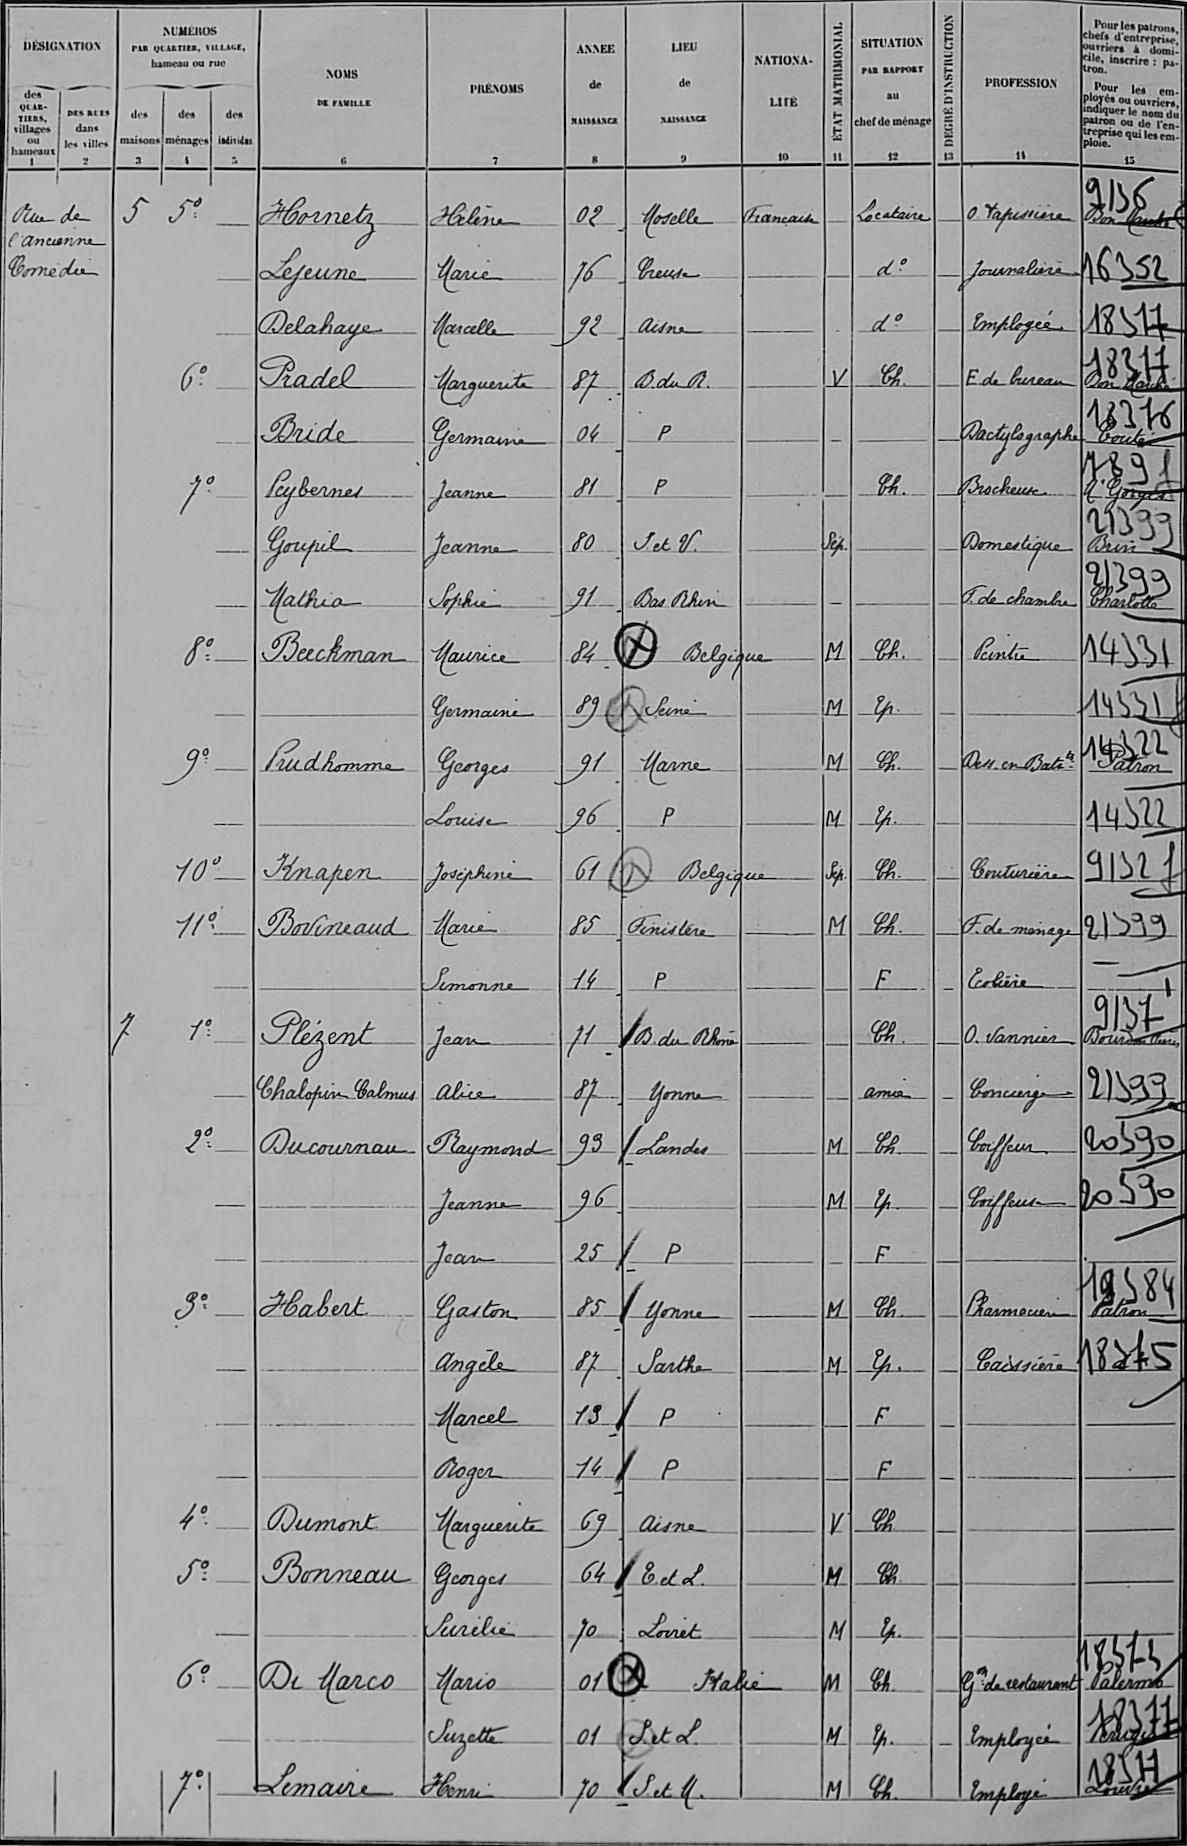Hornetz/Hélène/02/Moselle/française/¤/Locataire/¤/O Tapissière/Bon Marché?9136
Lejeune/Marie/76/Creuse/¤/¤/d°/¤/Journalière/16352
Delahaye/MArcelle/92/Aisne/¤/¤/d°/¤/Employée/18377
Pradel/Marguerite/87/B du R /¤/V/Ch /¤/E de bureau/Bon Marché?18377
Bride/Germaine/04/P/¤/¤/¤/¤/Dactylographe/Couté?18376
Peybernes/Jeanne/81/P/¤/¤/Ch /¤/Brocheuse/M Georges?7891
Goupil/Jeanne/80/I et V /¤/Sép/¤/¤/Domestique/Brin?21399
Mathia/Sophie/91/BAs Rhin/¤/¤/¤/¤/F de chambre/Charlotte?21399
Berckman/Maurice/84/Belgique/¤/M/Ch /¤/Peintre/14331
¤/Germaine/89/Seine/¤/M/Ep /¤/¤/15331
Prudhomme/geroges/91/Marne/¤/M/Ch /¤/Dess en Batx/Patron?14322
¤/Louise/96/P/¤/M/Ep /¤/¤/14322
Knapen/Joséphine/61/Belgique/¤/Sep /Ch /¤/Couturière/91327
Bovineaud/Marie/85/Finistère/¤/M/Ch /¤/F de méange/21399
¤/Simonne/14/P/¤/¤/F/¤/Ecolière/¤
Plézent/Jean/71/B du Rhône/¤/¤/Ch /¤/O vannier/Bourdon frères?9137
Chalopon Calmus/Alice/87/Yonne/¤/¤/amie/¤/Concierge/21399
Ducournau/Raymond/93/Landes/¤/M/Ch /¤/Coiffeur/20390
¤/Jeanne/96/¤/¤/M/Ep /¤/Coiffeur/20590
¤/Jean/25/P/¤/¤/F/¤/¤/¤
Habert/Gaston/85/Yonne/¤/M/Ch /¤/Pharmacien/Patron?19384
¤/Angèle/87/Sarthe/¤/M/Ep /¤/Caissière/18375
¤/MArcel/13/P/¤/¤/F/¤/¤/¤
¤/Roger/14/P/¤/¤/F/¤/¤/¤
Dumont/Marguerite/69/Aisne/¤/V/Ch/¤/¤/¤
Bonnuea/Georges/64/E et L /¤/M/Ch /¤/¤/¤
¤/Surélie/70/Loiret/¤/M/Ep /¤/¤/¤
De Marco/MArio/01/Italie/¤/M/Ch /¤/gon de restaurant/Palermo?18373
¤/Suzette/01/S et L /¤/M/Ep /¤/Employée/Péruget?18377
Lemaire/Henri/70/S et M /¤/M/Ch /¤/Employée/Louvre?18377
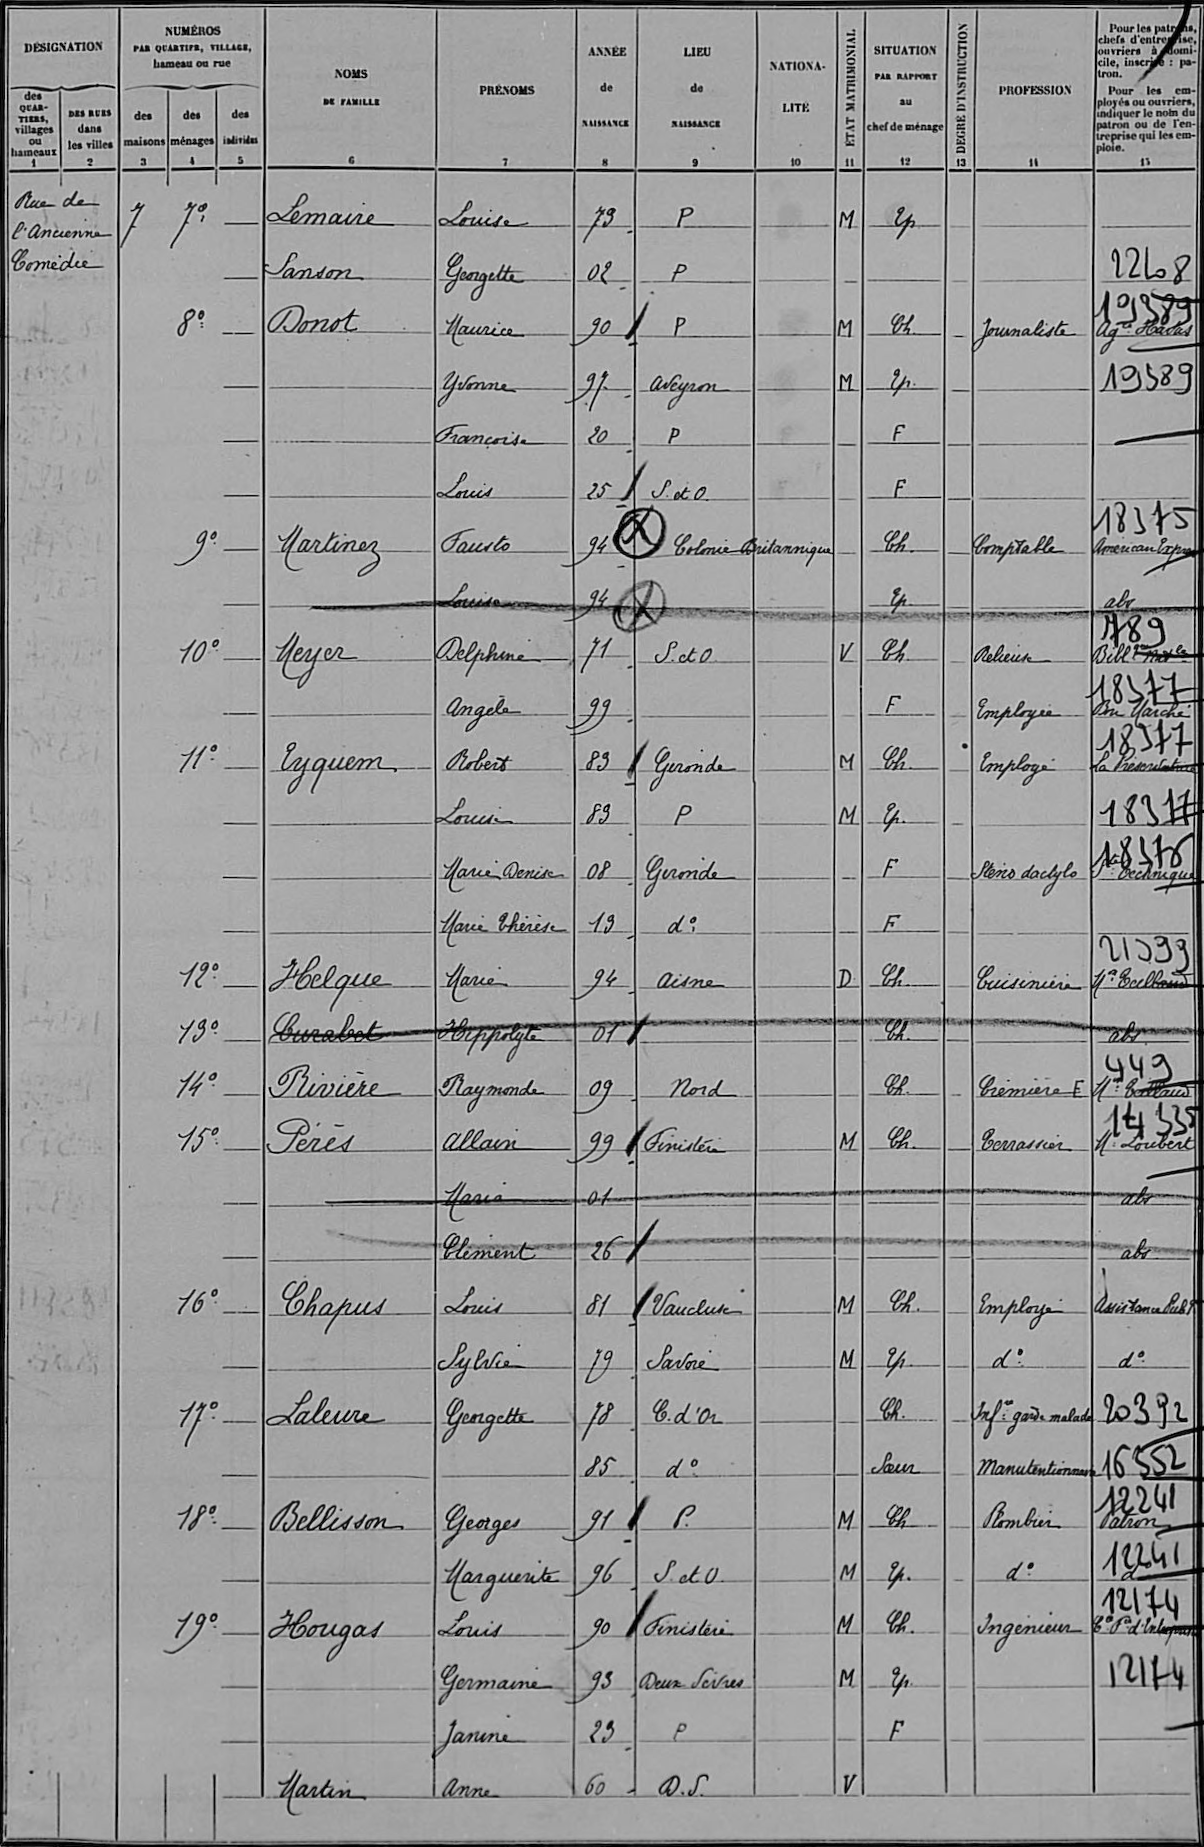Lemaire/Louise/73/P/¤/M/Ep/¤/¤/¤
Sanson/Georgette/02/P/¤/¤/¤/¤/¤/22408
Donot/Maurice/90/P/¤/M/Ch/¤/Journaliste/Agce Havas?19389
¤/Yvonne/97/aveyron/¤/M/Ep /¤/¤/19389
¤/Françoise/20/P/¤/¤/F/¤/¤/¤
¤/Louis/25/S et O /¤/¤/F/¤/¤/¤
Martinez/Fausto/94/Colonie Britannique/¤/¤/Ch /¤/Comptable/American Express?18375
¤/Louise/94/¤/¤/¤/Ep/¤/¤/abs
Meyer/Delphine/71/S et O /¤/V/ch/¤/Relieur/Bibl Natle?789
¤/Angele/99/¤/¤/¤/F/¤/Employee/Bon Marché?18377
Eyquem/Robert/83/Gironde/¤/M/Ch /¤/Employé/LaPrésentatice?18377
¤/Louise/83/P/¤/M/Ep/¤/¤/18377
¤/Marie Denise/08/Gironde/¤/¤/F/¤/Sténo dactylo/Ste technique?18376
¤/Marie Thérèse/13/d°/¤/¤/F/¤/¤/¤
Helque/Marie/94/aisne/¤/D/Ch/¤/Cuisinière/M Teillaud?21399
Lurabet/Hippolyte/01/¤/¤/¤/Ch /¤/¤/abs
Rivière/Raymonde/09/Nord/¤/¤/Ch /¤/Crémière E/M Teillaud?449
Pérès/Allain/99/Finistère/¤/M/Ch /¤/Terrassier/M Loubert?14335
¤/Maria/01/¤/¤/¤/¤/¤/¤/abs
¤/Clément/26/¤/¤/¤/¤/¤/¤/abs
Chapus/Louis/81/Vaucluse/¤/M/Ch /¤/Employé/Assistance Publiq
¤/Sylvie/79/Savoie/¤/M/Ep /¤/d°/d°
Laleure/Georgette/78/C d'Or/¤/¤/Ch /¤/Inf garde malade/20392
¤/¤/85/d°/¤/¤/Soeur/¤/Manutentionnaire/16352
Bellisson/Georges/91/P/¤/M/Ch/¤/Plombier/Patron?12241
¤/Marguerite/96/S et O /¤/M/Ep /¤/d°/d°?12241
Hougas/Louis/90/Finistère/¤/M/Ch /¤/Ingénieur/Cce F d'Entreprises?12174
¤/Germaine/93/Deux Sèvres/¤/M/Ep /¤/¤/12174
¤/Janine/23/P/¤/¤/F/¤/¤/¤
Martin/Anne/60/D S /¤/V/¤/¤/¤/¤